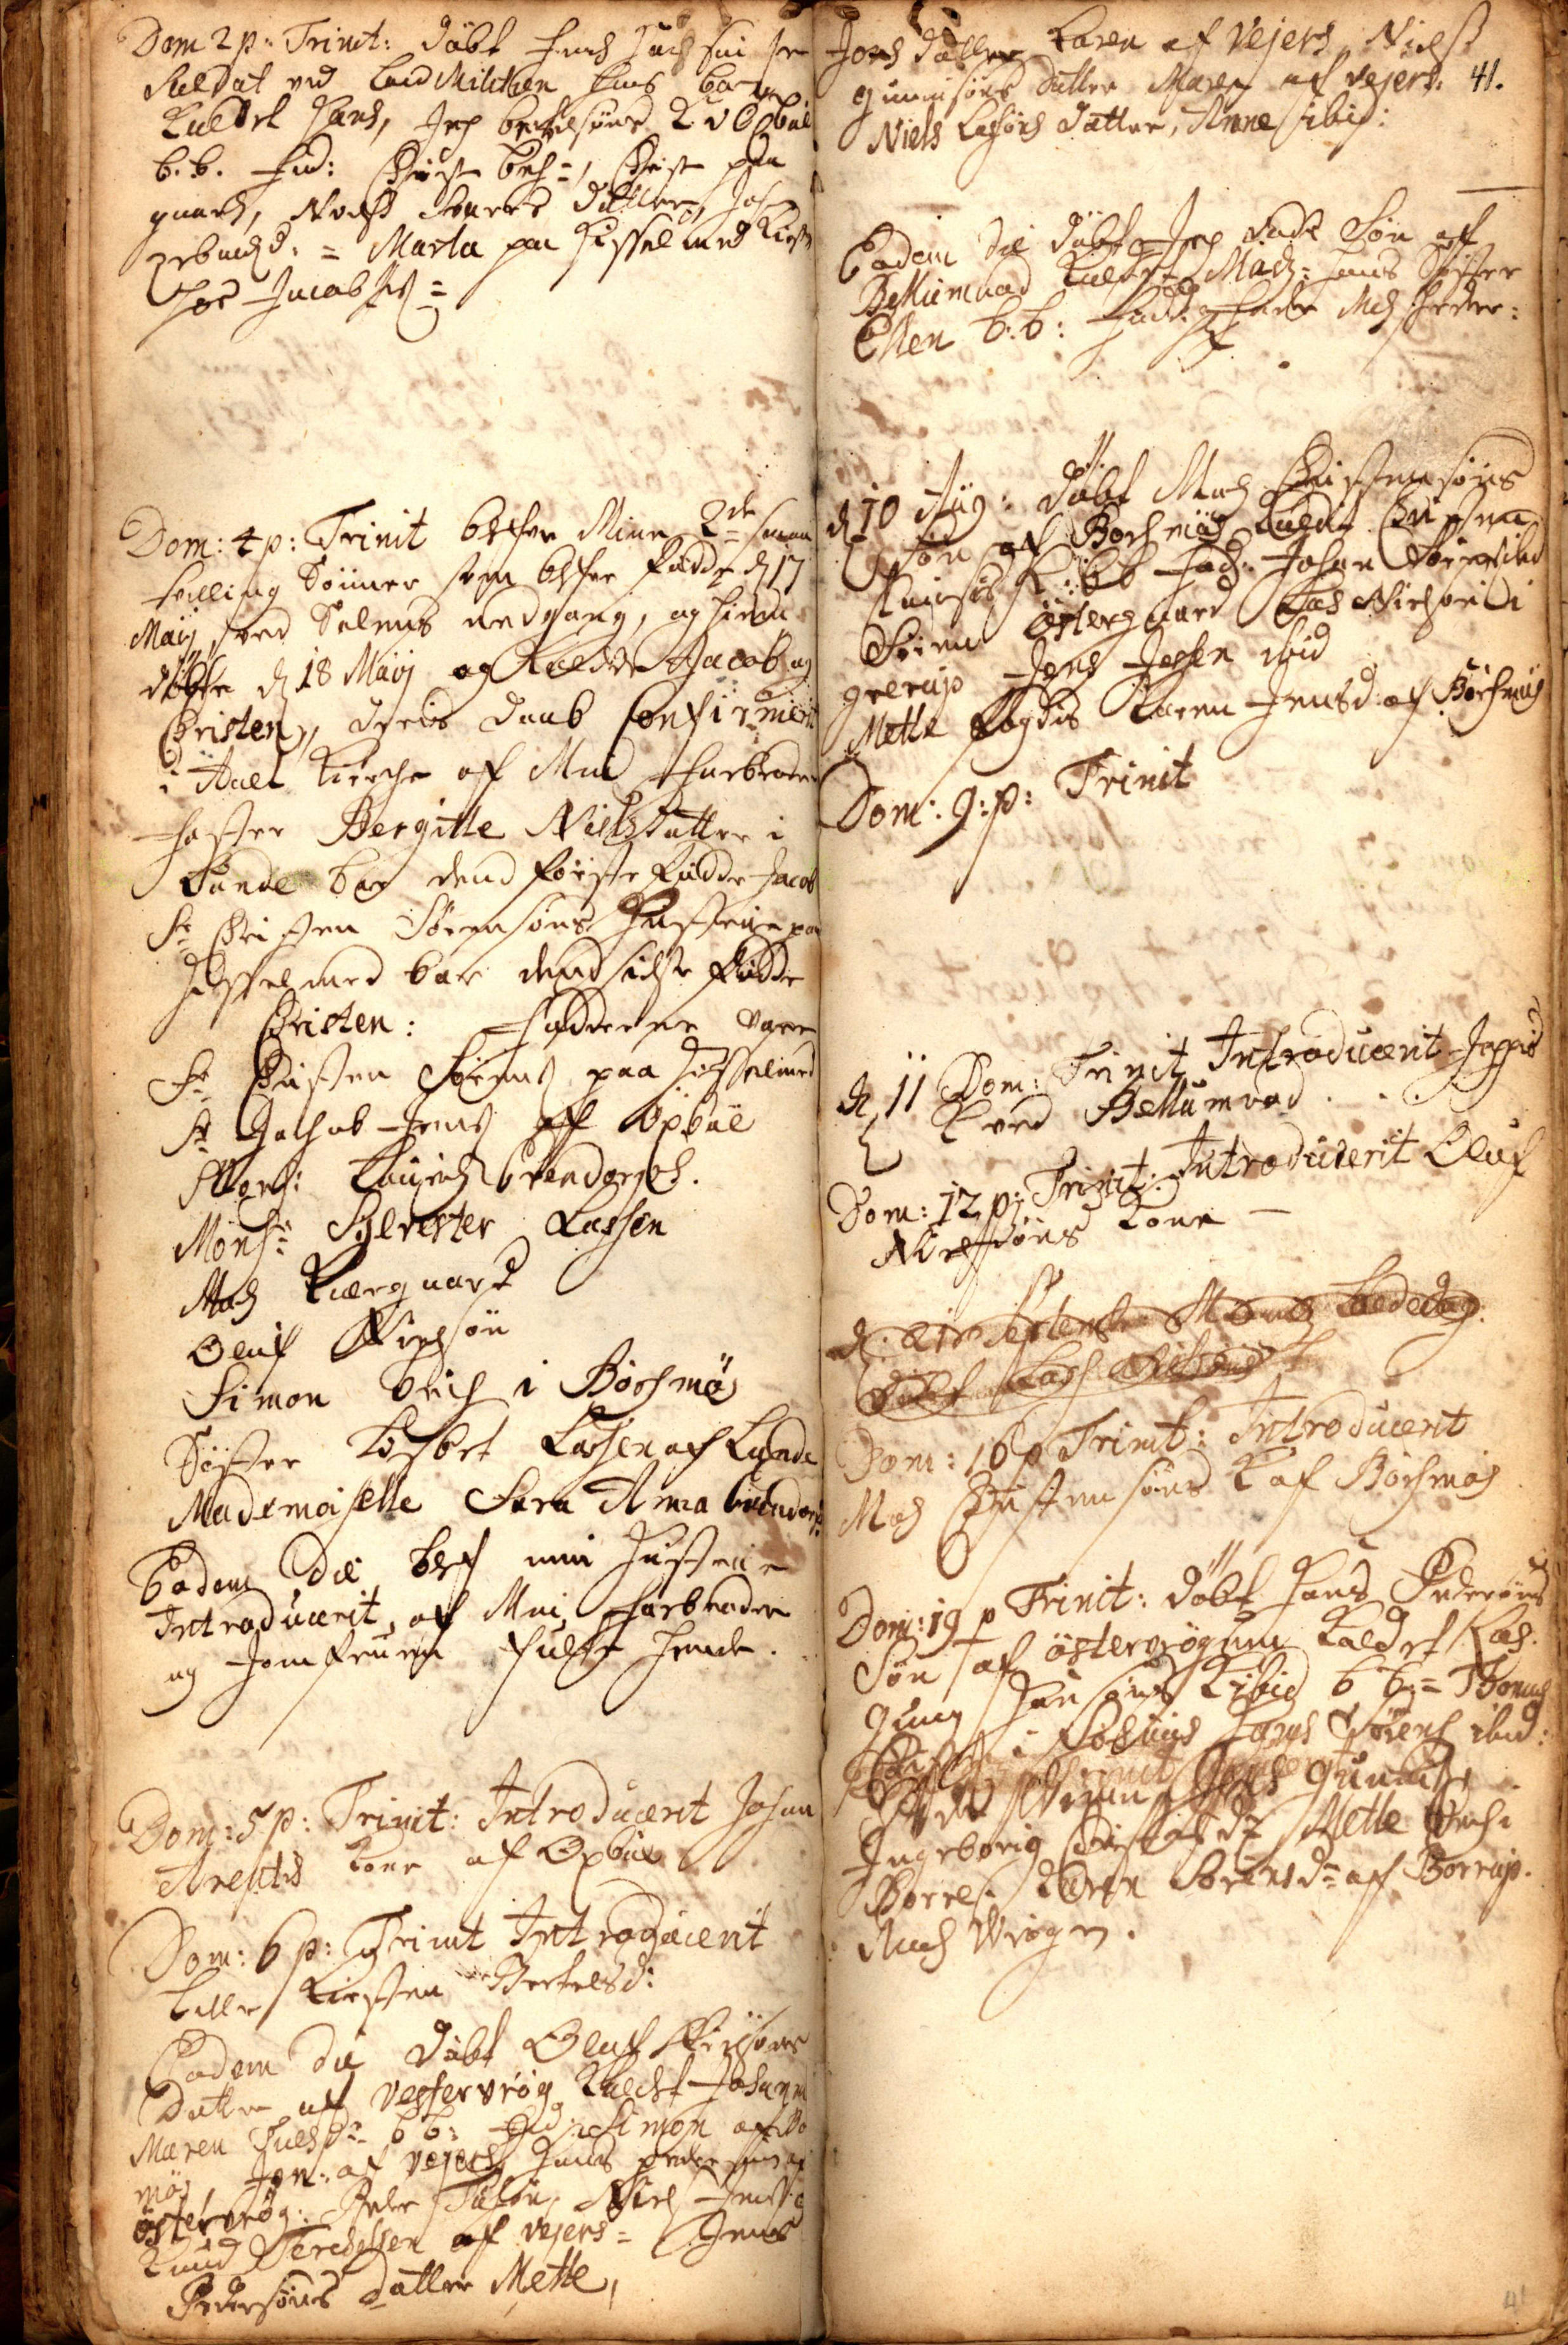Dom 2 p: Trinit: døbt Jens Huchsuis## ##
Soldat ved Land Militsen hans Barn
kaldet Hans, Jep Bertelsøns K i Oxbøl
b..b. Fad: Christen Brich=, Christen paa
gaard, Niels Svarres Datter, Johanne
Zebae d:= Marta paa Hisselmed, Kirsten
hos Jacob Jens=
Dom: 4 p: Trinit blefve Mine 2de smaa
Tvilling Sønner som blefve fødde d 17
Maij ved Solens nedgang, og hiemme
døbte d 18 Maij og kaldte Jacob og
Christen, deris Daab Confirmert
i Aael Kirche af Min Farbroder
Faster Bergitte Nielsdatter i
Lunde bar dend første fødde Jacob
Sr Christen Sørensøns Hustrue paa
Hisselmed bar dend sidste fødde
Christen: Fadderne vare
Sr Christen Sørens paa Hisselmed,
Sr Jachob Jens af Oxbøl,
Mons: Lauridz Brendorph.
Monsr Silvester Lassen
Madz Kiærgaard
Oluf Nielsøn
Simon Brich i Børsmøs,
Søster Lisbet Lassen af Lunde
Mademoiselle Sara Anna Brendorph.
Eodem Die blef min Hustrue
Introducerit af min Farbroder
og Jomfruen fulgte hende
Dom: 5 p: Trinit: Introducerit Johan
Arentis Kone af Oxbøl
Dom: 6 p: Trinit. Introducerit
Lille Kirsten Bertelsd:
Eodem Die Døbt Oluf Nielsøns
Datter af Vestervrøg kaldet Johanne
Maren Tuesdr b b: Fad: Simon af Bo
møs, Jon:  af Vejers, Hans Pedersen af
Østervrøg: Peder Tusøn, Niels Jens ##
Knud Terchelsen af Vejers= Jens
Pedersøns Datter Mette,
41
41
Jons Datter Karen af Vejers, Niels
Gunnisøns Datter Maren af Vejers:
Niels Lassøns Datter, Anne ibid:
Eodem Die døbt Jep Vads Søn af
Bellumvad kaldet Madz= Hans Søster
Ellen b:b: Fadd## Madz Schreder.:
D 10 Aug: døbt Madz Christensøns
Søn af Børsmøs kaldit Christen
Clausis K: bb Fad: Johan Sørensen ibd
Søren Østergaard, Las Nielsøn i
Grerup, Jens Jessen ibid
Mette Fogdis Karen Jensd: af Børsmøs.
Dom: 9: p: Trinit
D 11 Dom: Trinit Introducerit Jeppis
Kone Billumvad.
Dom:.12 p: Trinit: Introducerit Oluf
Nielsøns Kone-
d: 1 Septembr: Maanbeds Bededag:
døbt Las ##
Dom: 16 p Trinit:  Introducerit
Madz Christensøns K af Børsmøs
Dom: 19 p Trinit: døbt Hans Pedersøns
Søn af Østervrøgum kaldet Las.
Gunj Hansøns K ibid b b:=. Thomas
Christensen i Søhuus, Hans Sørensen ibid:
## ##
Peder Wium Jens Gunnis
Ingeborig Christensdr Mette Brich i
Borre. Karen Sørensdr af Borrup.
Madz Wrøg
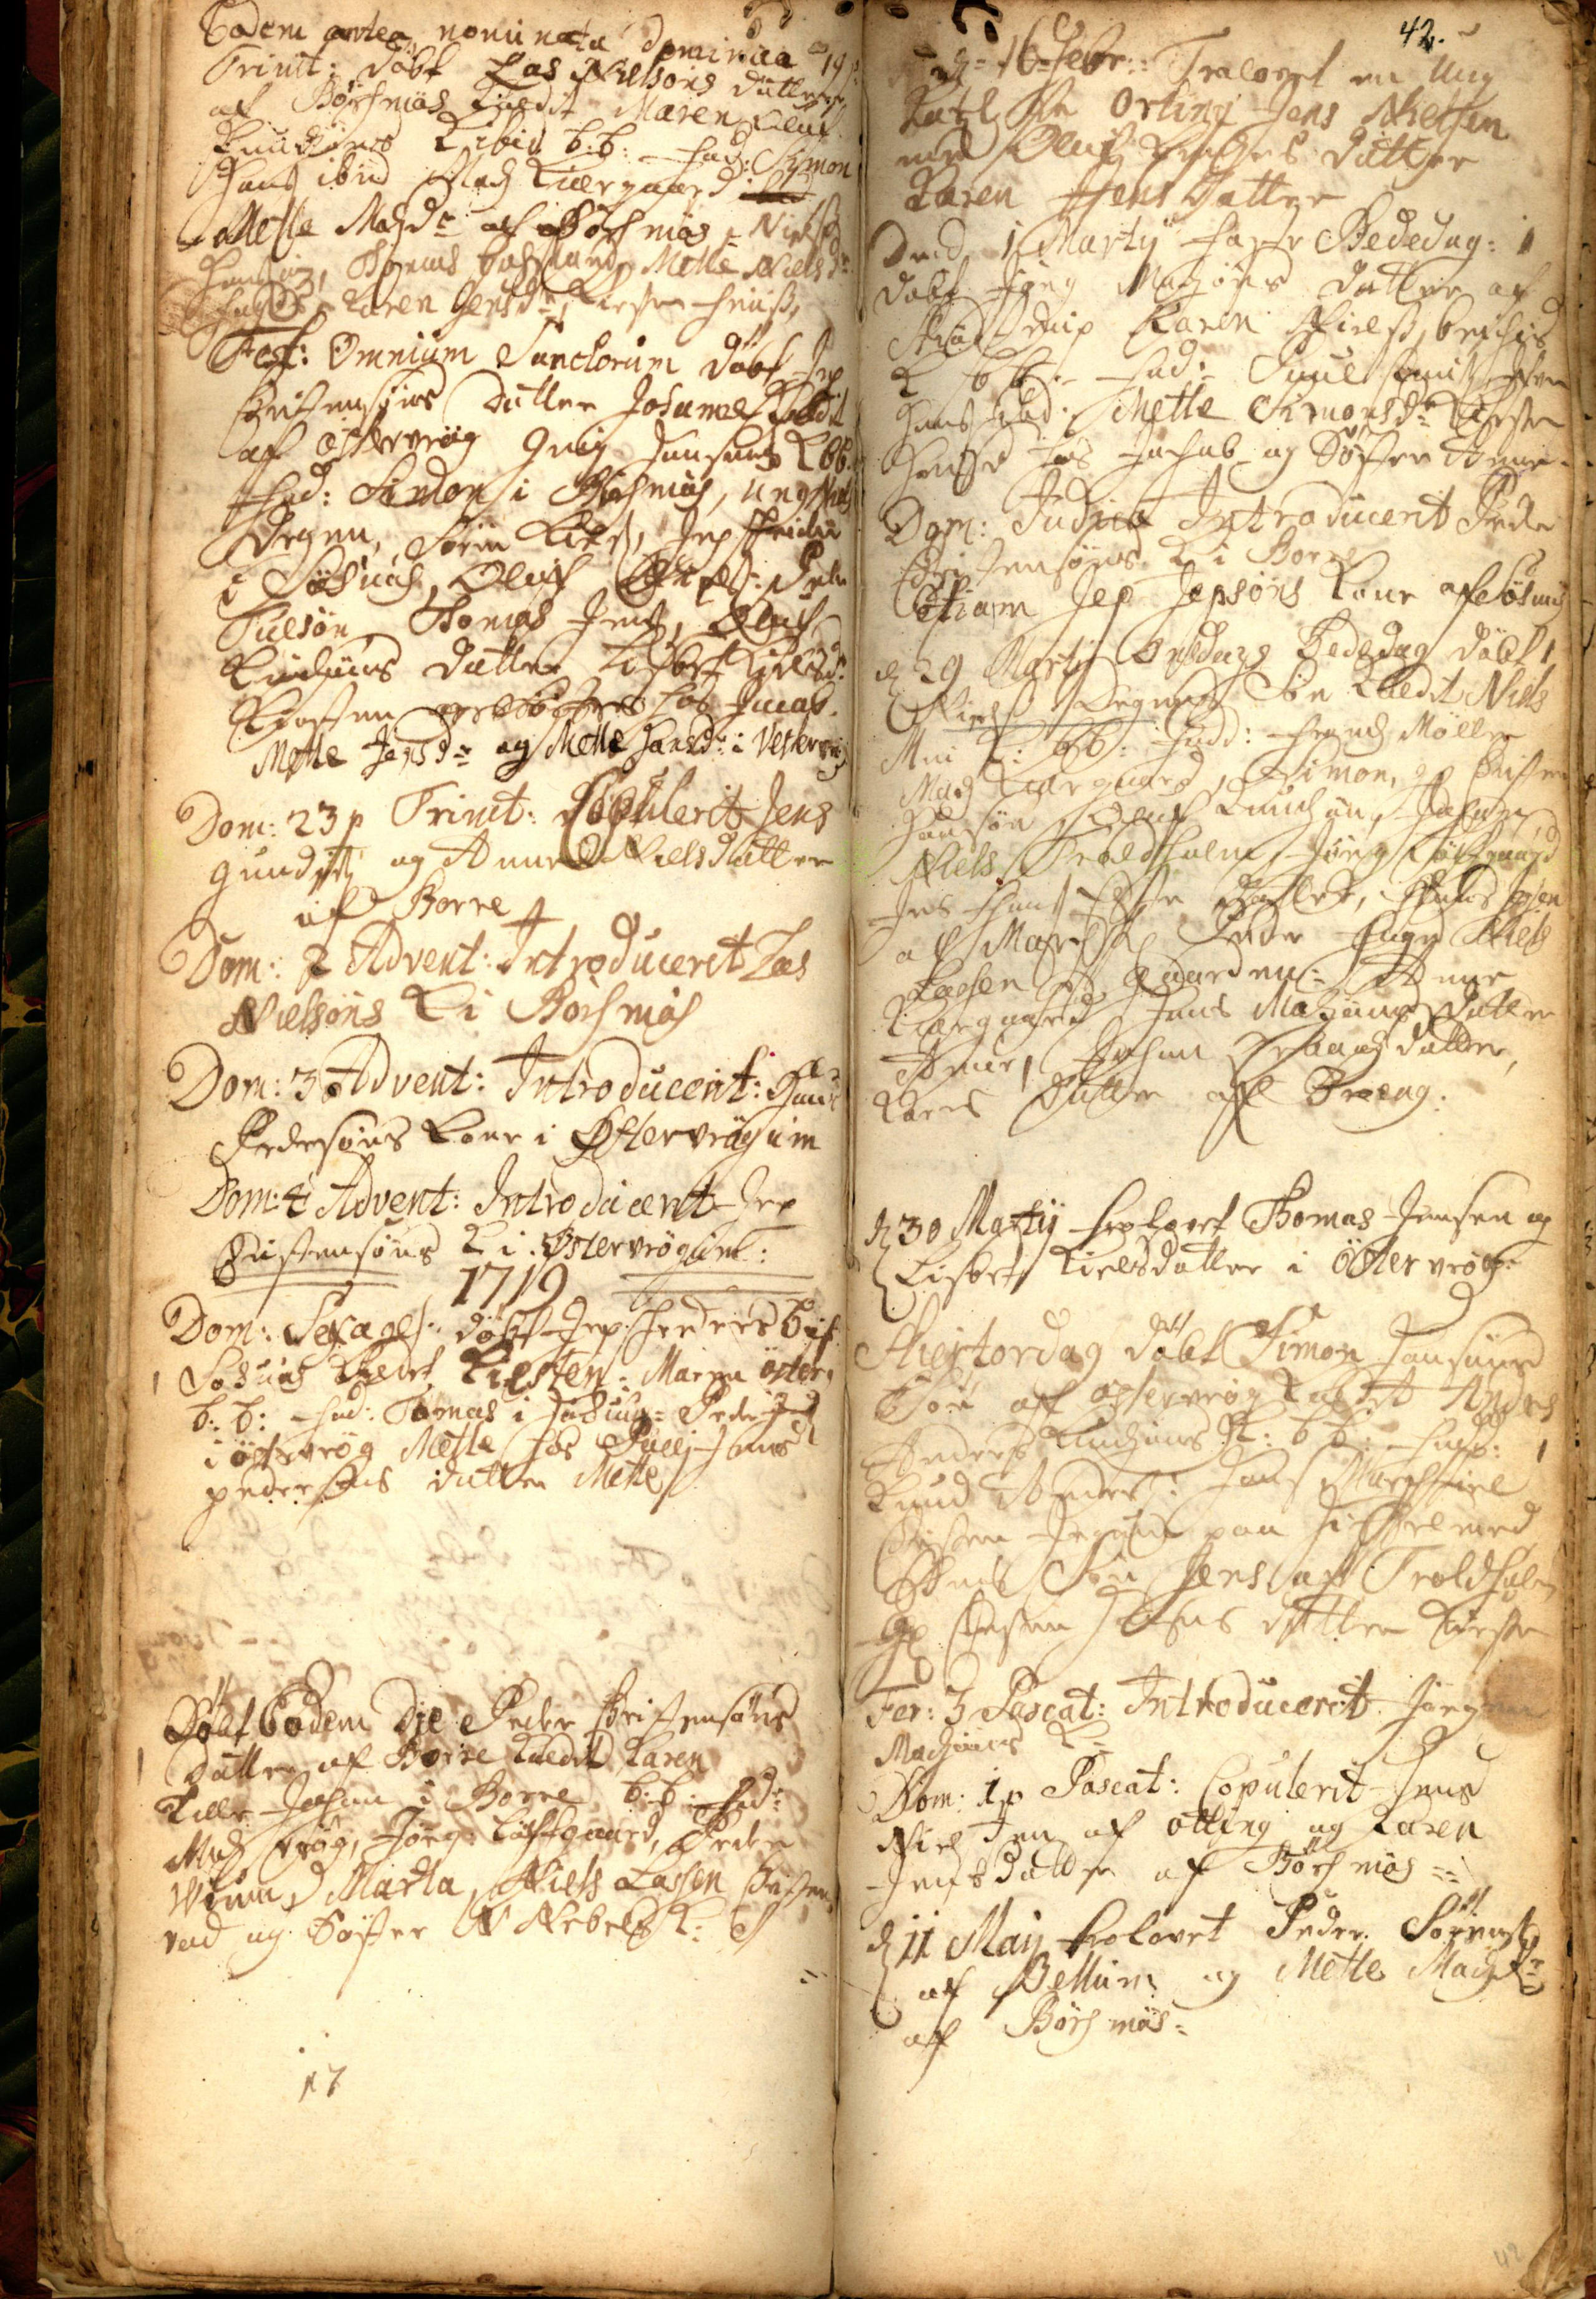Eodem antee## nominata Dominica 19 p:
Trinit: døbt Las Nielsøns Datter
af Børsmøs kaldit Maren Oluf
Knudzøns K ibid b:b: Fad: Simon
Hansen ibid Madz Kiærgaard ibid
Mette Madzdr af Børsmøs= Niels
Hans##, Thomas Bodz Mmand, Mette Nielsdr
Fogdis, Karen Jensdr, Kirsten Friis,
Fest: Omnium Sanctorum døbt Jep
Christensøns Datter Johanne kaldit
af Østervrøg Gunj Hansens K bb.
Fad: Simon i Børsmøs, Ung Niels
Degen, Søren Kiels, Jep Schreder
i Søhuus Oluf ##s: Peder
Tuesøn, Thomas Jens Oluf
Knudzøns Datter Lisbet Kielsd.
Kirsten ## ## hos Jacob.
Mette Jensdr og Mette Hansdr i Vestervrøg
Dom: 23 p Trinit: Copulerit Jens
Gundis og Anne Nielsdatter
af Borre
Dom: 2 Advent: Introducerit Las
Nielsøns K i Børsmøs
Dom: 3 Advent: Introducerit Hans
Pedersøns Kone i Østervrøgum
Dom: 4 Advent: Introducerit Jep
Christensøns K i Østervrøgum:
1719
Dom: Sexages: Døbt Jep Schreders B af
Søhuus kaldet Kiesten: Maren Østerg
b: b: Fad: Thomas i Søhuus= Peder J##
i Østervrøg Mette hos Pallj Jens
Pedersens Datter Mette
Døbt Eodem Die Peder Christensøns
Datter af Borre kaldet Karen
Lille J## i Borre b.b. Fad:
Madz Vrøg, Jørg: Løftgaard, Peder
Wium, Marta, Niels Lassen Christen
Vad og Søster, N Nebels K:
42
42
D= 16= Febr::Trolovet en Ung
Karl fra Orting Jens Nielsen
med Oluf Knudzøns Datter
Karen Jensdatter
Dend 1 Martij Faste Bededag: ##
døbt Jørg Madzøns Datter af
Skiødstrup Karen Niels Brichis
K bb: Fad: Poul Christensen, Ifver
Hans ibid: Mette Simonsdr Kirsten
Hansd hos Jachob og Søster Anne
Dom: Judica Introducerit Peder
Christensøns K i Borre
etiam Jep Jepsøns Kone af Søhuus
D 29 Martij Onsdags Bededag døbt ##
Niels Degens Søn kaldet Niels
Min K: bb: Fadd: Frandz Møller
Madz Kiærgaard, Simon, gl Christen
Hansøn, Oluf Knudzøn, ##
Niels Troldholm, Jørg Løftgaard
Jens Christensens Datter, Hans Jessen##
af Marchs## Peder ##gn Niels
Lassen p Gaarden=  Anne
Kiærgaard Jens Madzøns Datter
Anne, Johanne Zebæuz Datter
##s Datter af Broeng
D 30 Martij trolovet Thomas Jensen og
Lisbet Kielsdatter i Østervrøg:
Skiertorsdag døbt Simon Hansøns
Søn af Østervrøg kaldet Anders
Anders Knudzøns K: bb: Fadd: ##
Knud Anders: Hans Marchschiel
Christen Jegum paa Hisselmed
Brichs## Søn Jens af Troldholm
gl Christen Hans Datter Kirsten
Fer: 3 Pascat: Introducerit Jørgen
Madzøns K=
Dom: 1 p Pascat: Copulerit Jens
Nielsen af Otting og Karen
Jensdatter af Børsmøs==
D 11 Maij trolovet Peder Sørens
af Bellum og Mette Madzdr
af Børsmøs=